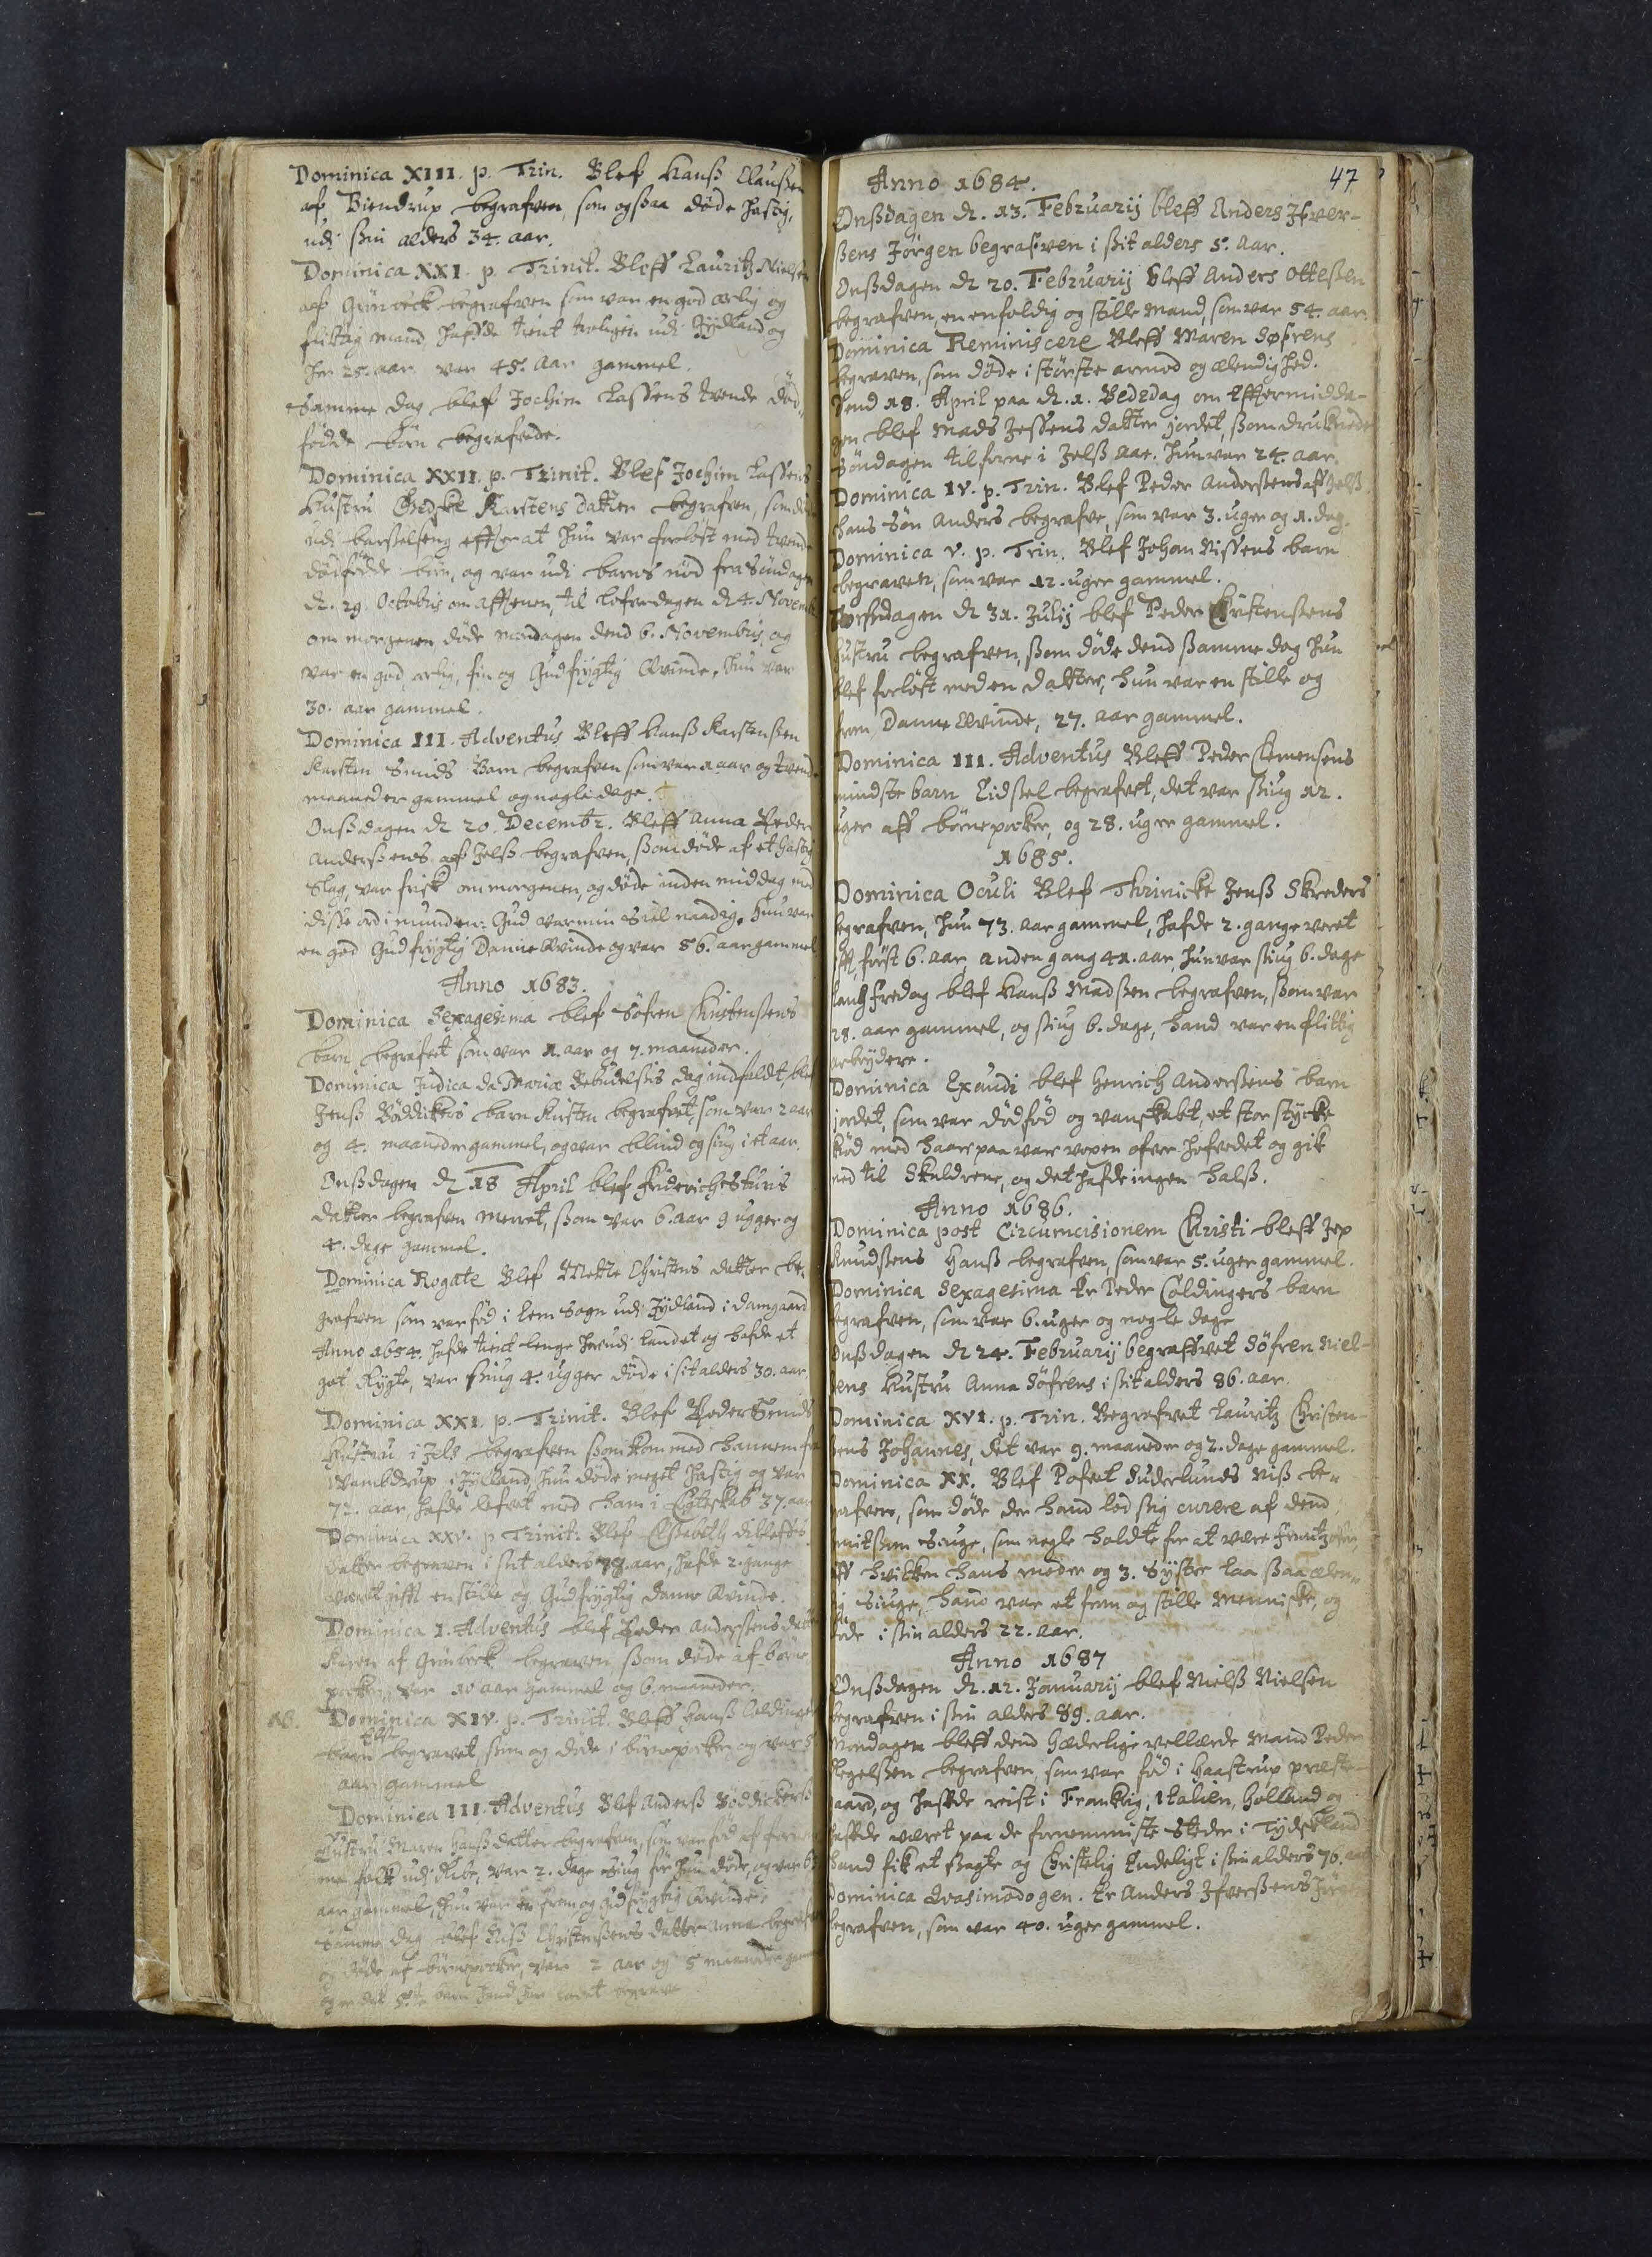Dominica XIII p. Trin. Blef Hans Claussen
af Biendrup begrafven, som ogssaa døde hastig,
udi ssin alders 34. aar.
Dominica XXI p. Trinit. Bleff Lauritz Nielsen
aff Grønbeck begrafven, som var en god arlig og
flittig mand, hafde tient troligen udi Jydland og
her 25. aar, var 45. Aar gammel.
Samme dag bleff Jochim Lassens tvende død¬
fødde børn begrafvede.
Dominica XXII p. Trinit. Blef Jochim Lassens
Hustru Gedske Karstens datter begrafven, som døde
udi barselseng efter at hun var forløst med hendis
dødfødde børn, og var udi barns nød fra Søndagen
d. 29 Octobris om afftenen til lofverdagen d 4. Novembris
om morgenen døde mandagen dend 6. Novembris, og
var en god, arlig, fin og Gudfrygtig Qvinde, hun var
30 aar gammel
Dominica III Adventus Bleff Hanss Karstenssen
Karsten Smids barn begrafven som var 1 aar og tvende
maaneder gammel og nogle dage.
Onssdagen d 20 Decembr. Bleff Anna Peder
Andersens aff Jelss begrafven, ssom døde af et hastig
slag, var frisk om morgenen, og døde inden middag med
disse ord i munden: Gud var min Siel naadig. Hun var
en god Gudfrygtig Danne Qvinde og var 56. aar gammel
Anno 1683.
Dominica Sexagesima blef Søfren Christensens
barn begrafvet som var 1. aar og 7. maaneder.
Dominica Judica da Maria Bebudelssis dag indfaldt blef
Jens Bøddikers barn Kirsten begrafvet, som var 2 aar
og 4. maaneder gammel, og var blind og siug i et aar.
Onssdagen d 18 April blef Friderich Sturis
datter begrafven Merret, ssom var 6 aar 9 ugger og
4. dage gammel.
Dominica Rogate Blef Mette Christens datter be¬
grafven som var fød i Lem Sogn udi Jydland i Damgaard
ANNO 1654. Hafde tient lenge herudi landet og hafde et
got Rygte, var siug 4. ugger døde i sit alders 30. aar.
Dominica XXI p. Trinit. Blef Peder Smids
Hustru i Jels begrafven ssom kom med hannem fra
Vamldrup i Jylland, hun døde meget hastig og var
72. aar, hafde lefvet med ham i Egteskab 37 aar
Dominica XXV. p. Trinit: Blef Elssebeth Ditleffs
datter begraven i sit alders 78. aar, hafde 2. gange
været gifft en stille og Gudfrygtig Danne Qvinde.
Dominica 1. Adventus blef Peder Andersens datter
Karen af Grønbeck begraven ssom døde af børne¬
pocker var 10 aar gammel og 6 maaneder.
NB. Dominica XIV p. Trinit Bleff Hans Coldingers
Else
barn begravet som og døde i burup k## og var 5
aar gammel
Dominica III Adventus Bleff Anderss Bøddikers
Hustru Maren Hanss datter begrafven, som var fød af
me folk udi Ribe, var 2. dage siug før hun døde, og var 63.
aar gammel, hun var en from og gudfrygtig qvinde.
Samme dag blef Niss Christenssens datter Anna begrafv 
og døde af børnepocker, var 2 aar og 5 maaneder gammel
og er det 5te barn hand har ladet begrave
47
Anno 1684
Onssdagen d 13. Februarij bleff Anders Ifver¬
sens Jørgen begrafven i ssit alders 5. Aar.
Onssdagen d 20. Februarij bleff Anders Ottessen
begrafven, en enfoldig og stille Mand, som var 54. aar.
Dominica Reminiscere Bleff Maren Søfrens
begraven, som døde i største armod og ælendighed.
Dend 18. April paa d. 1. Bededag om eftermidda¬
gen bleff Mads Jessens datter jordet, ssom druknede
Søndagen tilforne i Jelss aae. Hun var 24. aar.
Dominica IV. p. Trin. Blef Peder Andersens aff Jelss,
hans Søn Anders begrafve, som var 3. uger og 1. dag.
Dominica V. p. Trin. Blef Johan Nissens barn
begraven, som var 12. uger gammel.
Torsdagen d 31. Julij blef Peder Christensens
hustru begrafven, ssom døde dend ssamme dag hun
blef forløst med en datter, hun var en stille og
from Danne Qvinde, 27. aar gammel.
Dominica III. Adventus Bleff Peder Clemensens
mindste barn Cidsel begrafvet, det var ssiug 12.
uger aff børnepocker, og 28. uger gammel.
1685.
Dominica Oculi Blef Thrinicke Jenss Skreders
begrafven, hun 73. Aar gammel, hafde 2. gange veret
fft, først 6. aar anden gang 41. aar. hun var siug 6. dage
Langfredag blef Hanss Madssen begrafven, ssom var
28. aar gammel, og siug 6. dage, hand var en flittig
arbeydere
Dominica Exaudi blef Henrich Anderssens barn
jordet, som var dødfød og vanskabt, et stor stycke
kiød med haar paa var voxen ofver hofvedet og gik
ned til Skuldrene, og det hafde ingen Halss.
Anno 1686.
Dominica post Circumcisionem Christi bleff Jep
Knudssens Hanss begrafven, som var 5. uger gammel.
Dominica Sexagesima Er Peder Coldingers barn
begrafven, som var 6. uger og nogle dage
Onsdagen d 24. Februarij begraffvet Søfren Niel¬
sens Hustru Anna Søfrens i ssit alders 86. aar.
Dominica XVI. p. Trin. Begrafvet Lauritz Christen¬
sens Johannes, det var 9. maaneder og 2. dage gammel.
Dominica XX. Blef Pofvel Suderlunds Niss be¬
afven, som døde der hand lod ssig curere af dend
smitsom Siuge, som nogle holdte for at være fretzofm##
aff hvilken hans moder og 3. Syster laa ssaa ælen¬
dig siuge, hand var et from og stille menniske, og
døde i sin alders 22. aar.
Anno 1687
Onssdagen d. 12. Januarij blef Nielss Nielsen
begrafven i ssin alders 89. aar.
Mandagen bleff dend hæderlige vellærde mand Peder
Regelssen begrafven, som var fød i Haastrup præste¬
aard, og hafde reist i Frankrig, Italien, Holland og
haffde været paa de fornemmiste Steder i Tydskland.
Hand fik et ssagte og Christelig Endeligt i sin alders 76.
Dominica Qvasimodogen. Er Anders Ifverssens Jørgen
begrafven, som var 40. uger gammel.